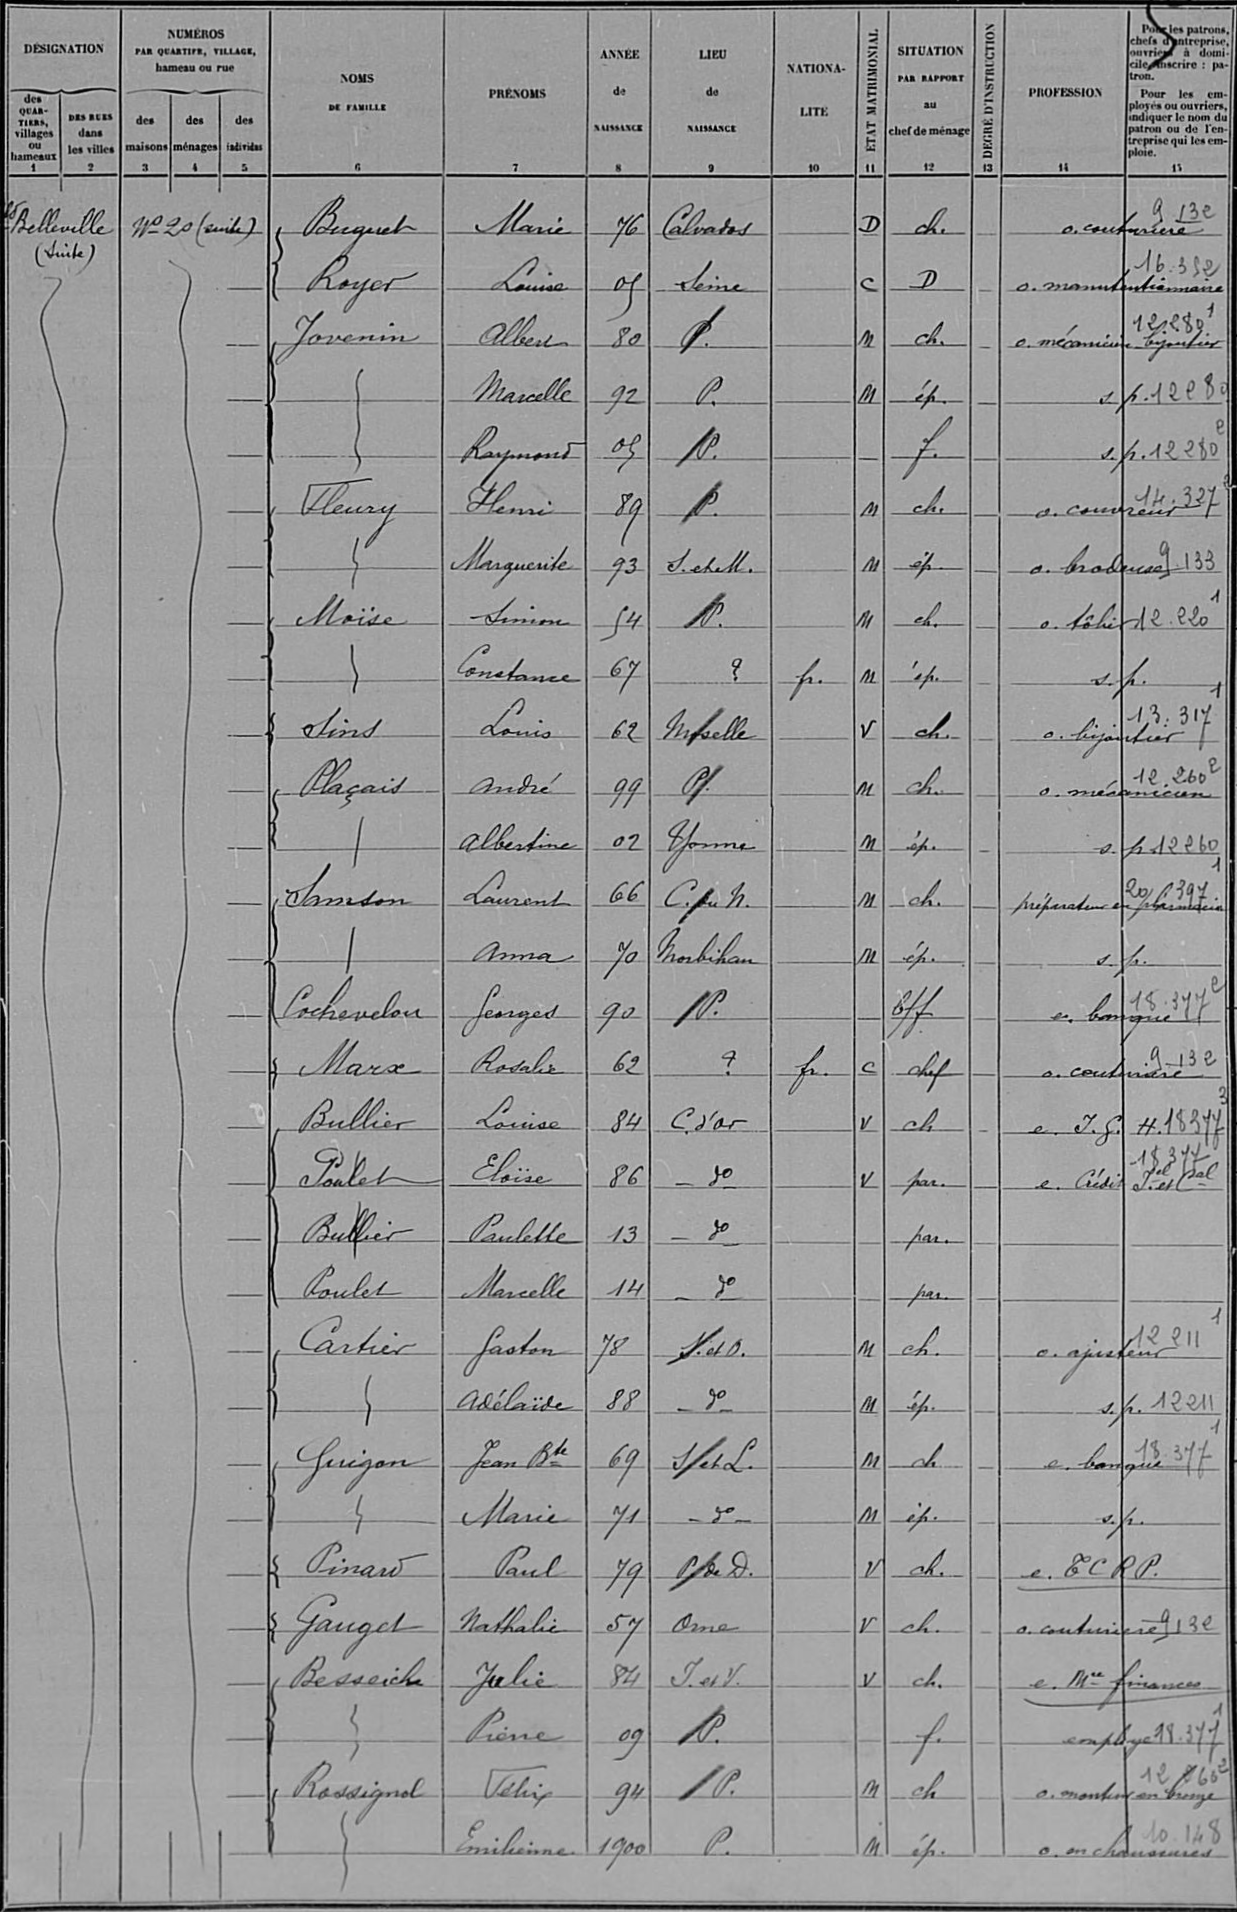Buguet/Marie/76/Calvados/¤/D/ch /¤/o couturiere/¤
Royer/Louise/05/Seine/¤/c/D/¤/o manutentionnaire/16 352
Jovenin/Albert/80/P /¤/M/ch /¤/o mécanicien/bijoutier
¤/Marcelle/92/P /¤/M/ép /¤/s/p 12280
¤/Raymond/05/P /¤/¤/f /¤/s /p
Fleury/Henri/89/P /¤/M/ch /¤/o couvreur/¤
¤/Marguerite/93/S et M /¤/M/ép/¤/o brodeuse/9133
Moïse/Simon/54/P /¤/M/ch /¤/o tôlier/122201
¤/Constance/67/¤/fr /M/ép /¤/s /pr
Sins/louis/62/Moselle/¤/V/ch /¤/o bijoutier/13 317
Plaçais/André/99/P /¤/M/ch /¤/o mécanicien/¤
¤/Albertine/02/Yonne/¤/M/ép /¤/s /p 122601
Samson/Laurent/66/C du N /¤/M/ch /¤/préparateur en pharmacie/¤
¤/Anna/70/Morbihan/¤/M/ép /¤/s /pr
Cochevelou/Georges/90/P /¤/¤/¤/¤/e banque/¤
Marx/Rosalie/62/¤/fr /c/chef/¤/o couturière/¤
Bullier/Louise/84/C d'or/¤/V/ch/¤/e I G /H 183773
Poulet/Eloïse/86/d°/¤/V/par /¤/e crédit/Iel et Cal
Bullier/Paulette/13/d°/¤/¤/par /¤/¤/¤/¤
Poulet/Marcelle/14/d°/¤/¤/par /¤/¤/1
Cartier/Gaston/78/S et O /¤/M/ch /¤/o ajusteur/122111
¤/Adélaïde/88/d°/¤/M/ép /¤/s /pr 122111
Guigon/Jean Bde/69/S et L /¤/M/ch/¤/e banque/¤
¤/Marie/71/d°/¤/M/ép /¤/s /pr
Pinard/Paul/79/P de D /¤/V/ch /¤/e TCR/P
Gauget/Nathalie/57/Orne/V/ch /¤/o couturière/9132
Besseich/Julie/84/I et V /¤/V/ch /¤/e mre finances/¤/¤
¤/Pierre/09/P /¤/¤/f /¤/employe/18 3771
Rossignol/Félix/94/P /¤/M/ch/¤/o mouleur en bronze/¤
¤/Emilienne/1900/P /¤/M/ép /¤/o en chaussures/¤
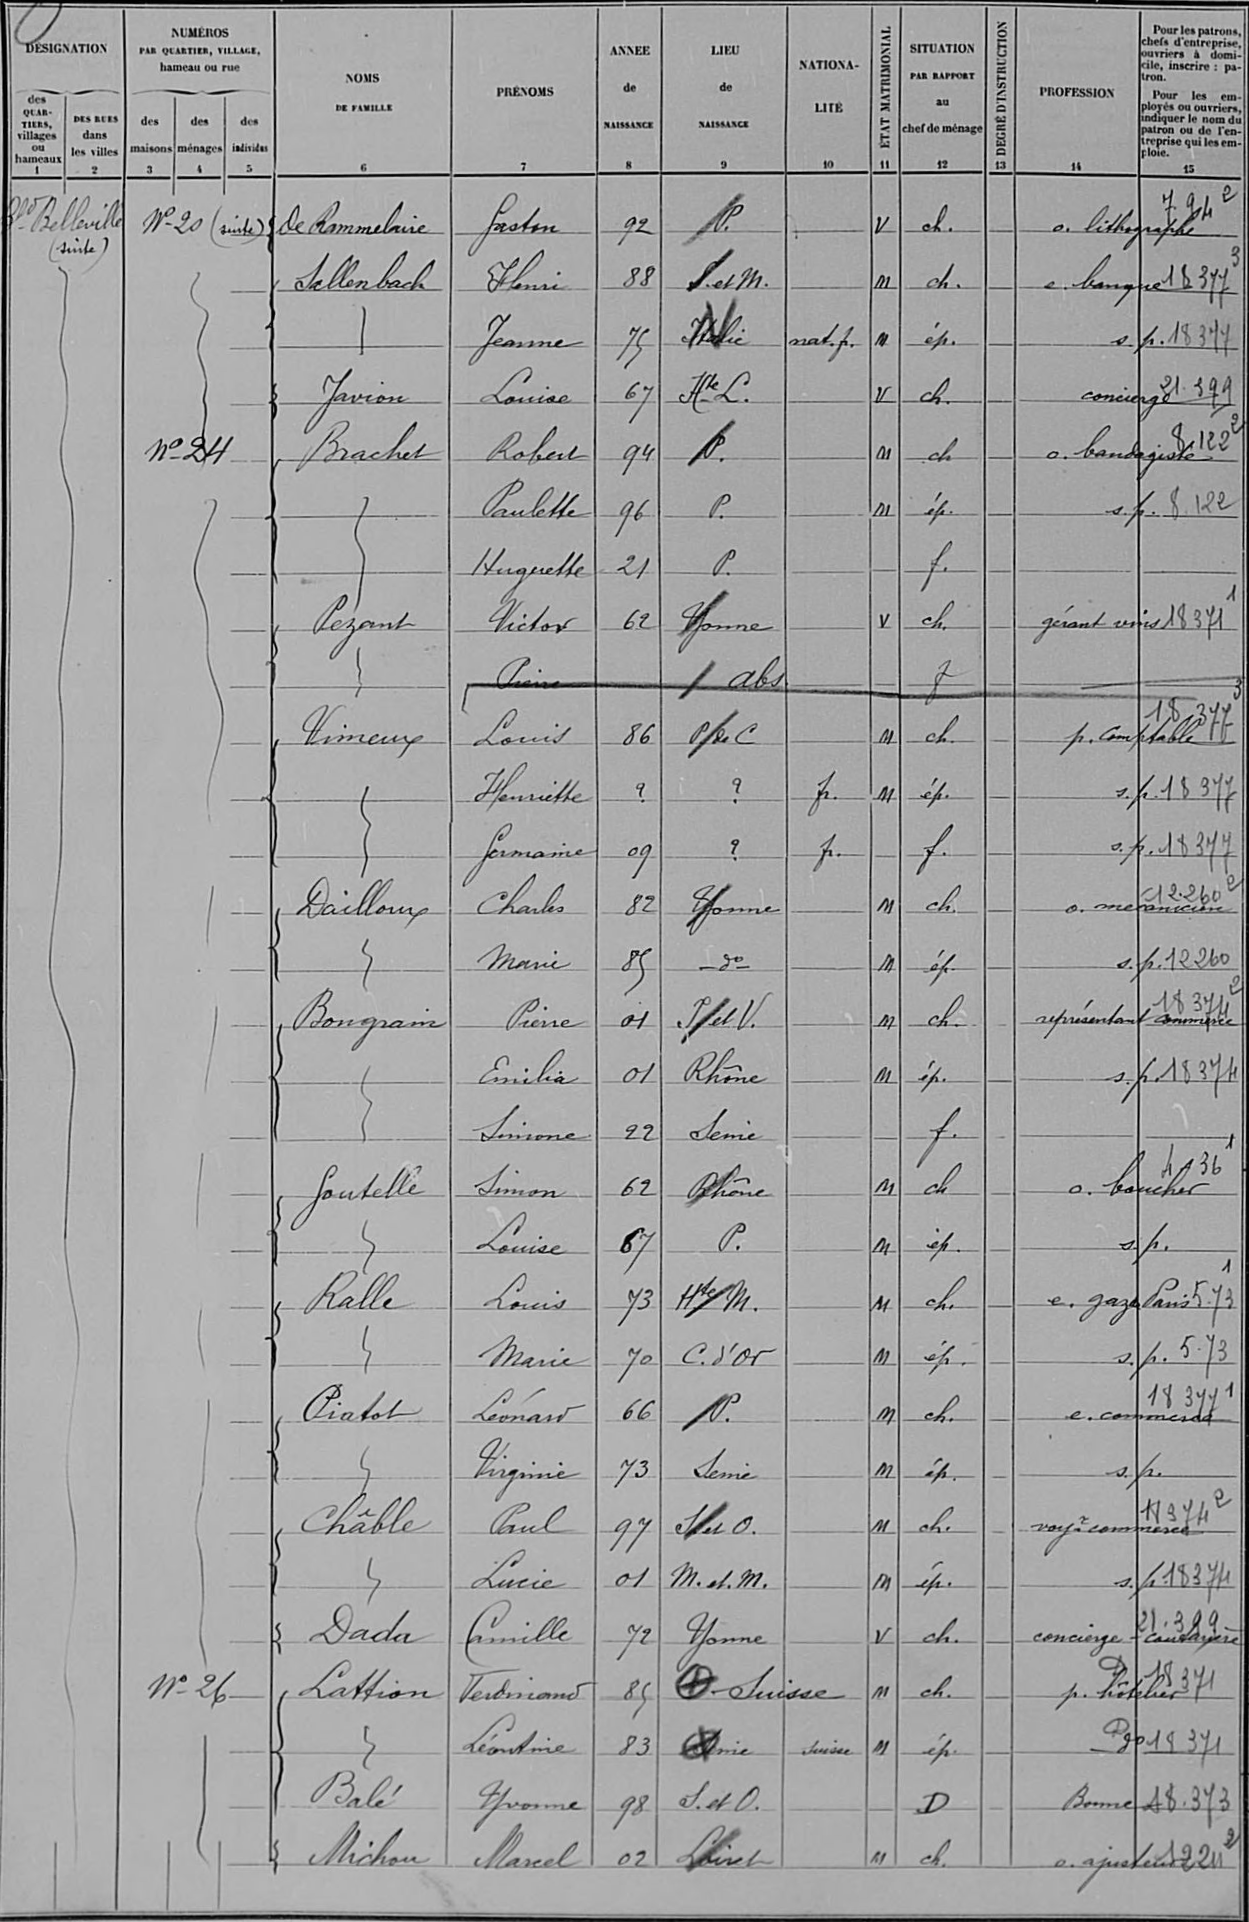de Rammelaire/Gaston/92/P /¤/V/ch /¤/o lithographe/794 2
Sellenbach/Henri/88/S et M /¤/m/ch /¤/e banque/183773
¤/Jeanne/75/Italie/nat fr/M/ép /¤/s /pr 18377
Javion/Louise/67/Hte L /¤/V/ch /¤/concierge/¤
Brachet/Robert/94/P /¤/M/ch/¤/o bandagiste/81222
¤/Paulette/96/P /¤/M/ép /¤/s p /8122
¤/Huguette/21/P /¤/¤/f /¤/¤/1
Pezant/Victor/62/Yonne/¤/V/ch /¤/gérant vins/18371
¤/Pierre/¤/abs/¤/¤/f/¤/¤/3
Vimeux/Louis/86/P de C/¤/M/ch /¤/p comptable/18377
¤/Henriette/¤/¤/fr /M/ép /¤/s /pr 18377
¤/Germaine/09/¤/fr /¤/f /¤/s /pr 18377
Dailloux/Charles/82/Yonne/¤/M/ch /¤/o mécanicien/¤
¤/Marie/85/d°/¤/M/ép/¤/s /pr /122602
Bougrain/Pierre/01/I et V /¤/m/ch /¤/représentant/commerce
¤/Emilie/01/Rhône/¤/M/ép /¤/s /p 18374
¤/Simone/22/Seine/¤/¤/f /¤/¤/1
Gouhelle/Simon/62/¤/Rhône/M/ch/¤/o boucher/4136
¤/Louise/67/P /¤/M/ép /¤/s /p /¤
Ralle/Louis/73/Hte M /¤/M/ch /¤/e gaz/Paris 5 73
¤/Marie/70/C d'Or/¤/M/ép /¤/s /p 5 73
Pialot/Léonard/66/P /¤/M/ch /¤/e commerce/¤
¤/Virginie/73/Seine/¤/M/ép /¤/s /pr /¤
Châble/Paul/97/S et O /¤/M/ch /¤/voyr-commerce/183742
¤/Lucie/01/M et M /¤/M/ép /¤/s /pr 18374
Dada/Camille/72/Yonne/¤/V/ch /¤/concierge-cuisinière
Lassion/Ferdinand/85/Suisse/¤/M/ch /¤/p hôtelier/¤
¤/Léontine/83/Seine/suisse/M/ép /¤/d°/18371
Balé/Yvonne/98/S et O /¤/¤/D/¤/Bonne/18373
Michou/Marcel/02/Loiret/¤/M/ch /¤/o ajusteur/122112
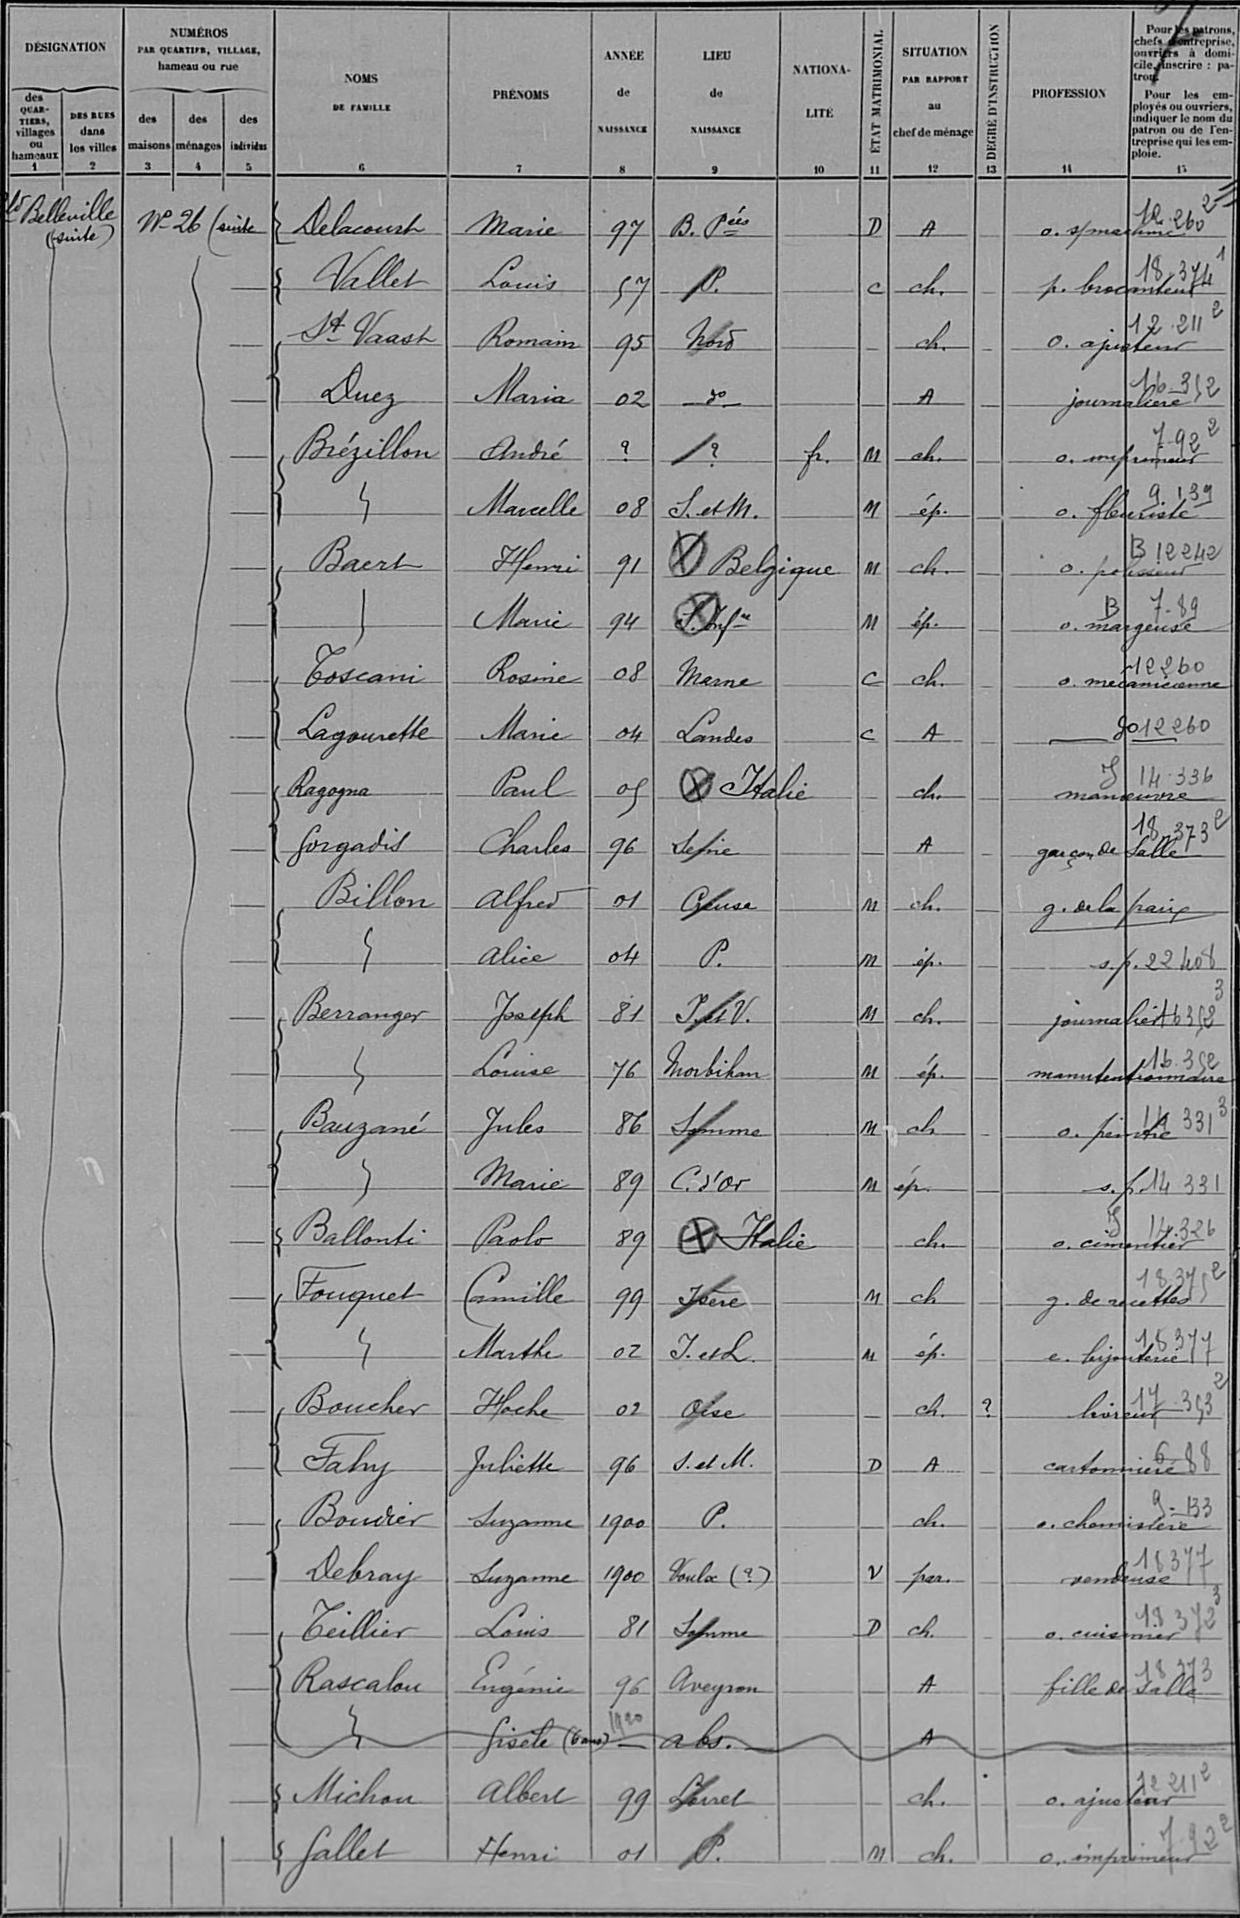Delacourt/Marie/97/B Pées/¤/D/A/¤/o smachine/122602
Vallet/Louis/57/P /¤/c/ch /¤/p brocanteur/¤
St Vaast/Romain/95/Nord/¤/¤/ch /¤/o ajusteur/¤
Duez/Maria/02/d°/¤/¤/A/¤/journaliere/16312
Brézillon/André/¤/¤/fr /M/ch /¤/o imprimeur/7922
¤/Marcelle/08/S et M /¤/M/ép /¤/o fleuriste/¤
Baert/Henri/91/Belgique/¤/M/ch /¤/o polisseur/¤
¤/Marie/94/S Infre/¤/M/ép /¤/o margeuse /¤
Toscani/Rosine/08/Marne/¤/c/ch /¤/o mécanicienne/¤
Lagourette/Marie/04/Landes/¤/C/A/¤/d°/12260
Ragogna/Paul/05/Italie/¤/¤/ch /¤/manoeuvre /¤
Gorgadis/Charles/96/Seine/¤/¤/A/¤/garçon de salle/¤
Billon/Alfred/01/Creuse/¤/M/ch /¤/g de la paix/¤/¤
¤/Alice/04/P /¤/M/ép /¤/s /p 22408
Berranger/Joseph/81/I et V /¤/M/ch /¤/journalier/163523
¤/Louise/76/Morbihan/¤/M/ép /¤/manutentionnaire/¤
Bauzané/Jules/86/Somme/¤/M/ch/o peintre/¤
¤/marie/89/C d'Or/¤/M/ép /¤/s /p 14331
Ballonhi/Paolo/89/Italie/¤/ch /¤/o cimentier/14 326
Fouquet/Camille/99/Isère/¤/M/ch/¤/g de recettes/¤
¤/Marthe/02/I et L /¤/M/ép/¤/e bijouterie/183772
Boucher/Hoche/02/Oise/¤/¤/ch /¤/livreur/17 3532
Fahy/Juliette/96/S et M /¤/D/A/¤/cartonnière/688
Bouvier/Suzanne/1900/P /¤/¤/ch /¤/o chemisière/9 133
Debray/Suzanne/1900/Voulx /¤/V/par /¤/vendeuse/¤
Ceillier/Louis/81/Somme/¤/D/ch /¤/o cuisinier/¤
Rascalou/Eugénie/96/Aveyron/¤/¤/A/¤/fille de salle/¤
¤/Gisèle (6 ans)/1930/abs /¤/¤/A/¤/¤/¤/¤
Michou/Albert/99/Loiret/¤/¤/ch /¤/o ajusteur/¤
Jallet/Henri/01/P /¤/m/ch /¤/o imprimeur/7922